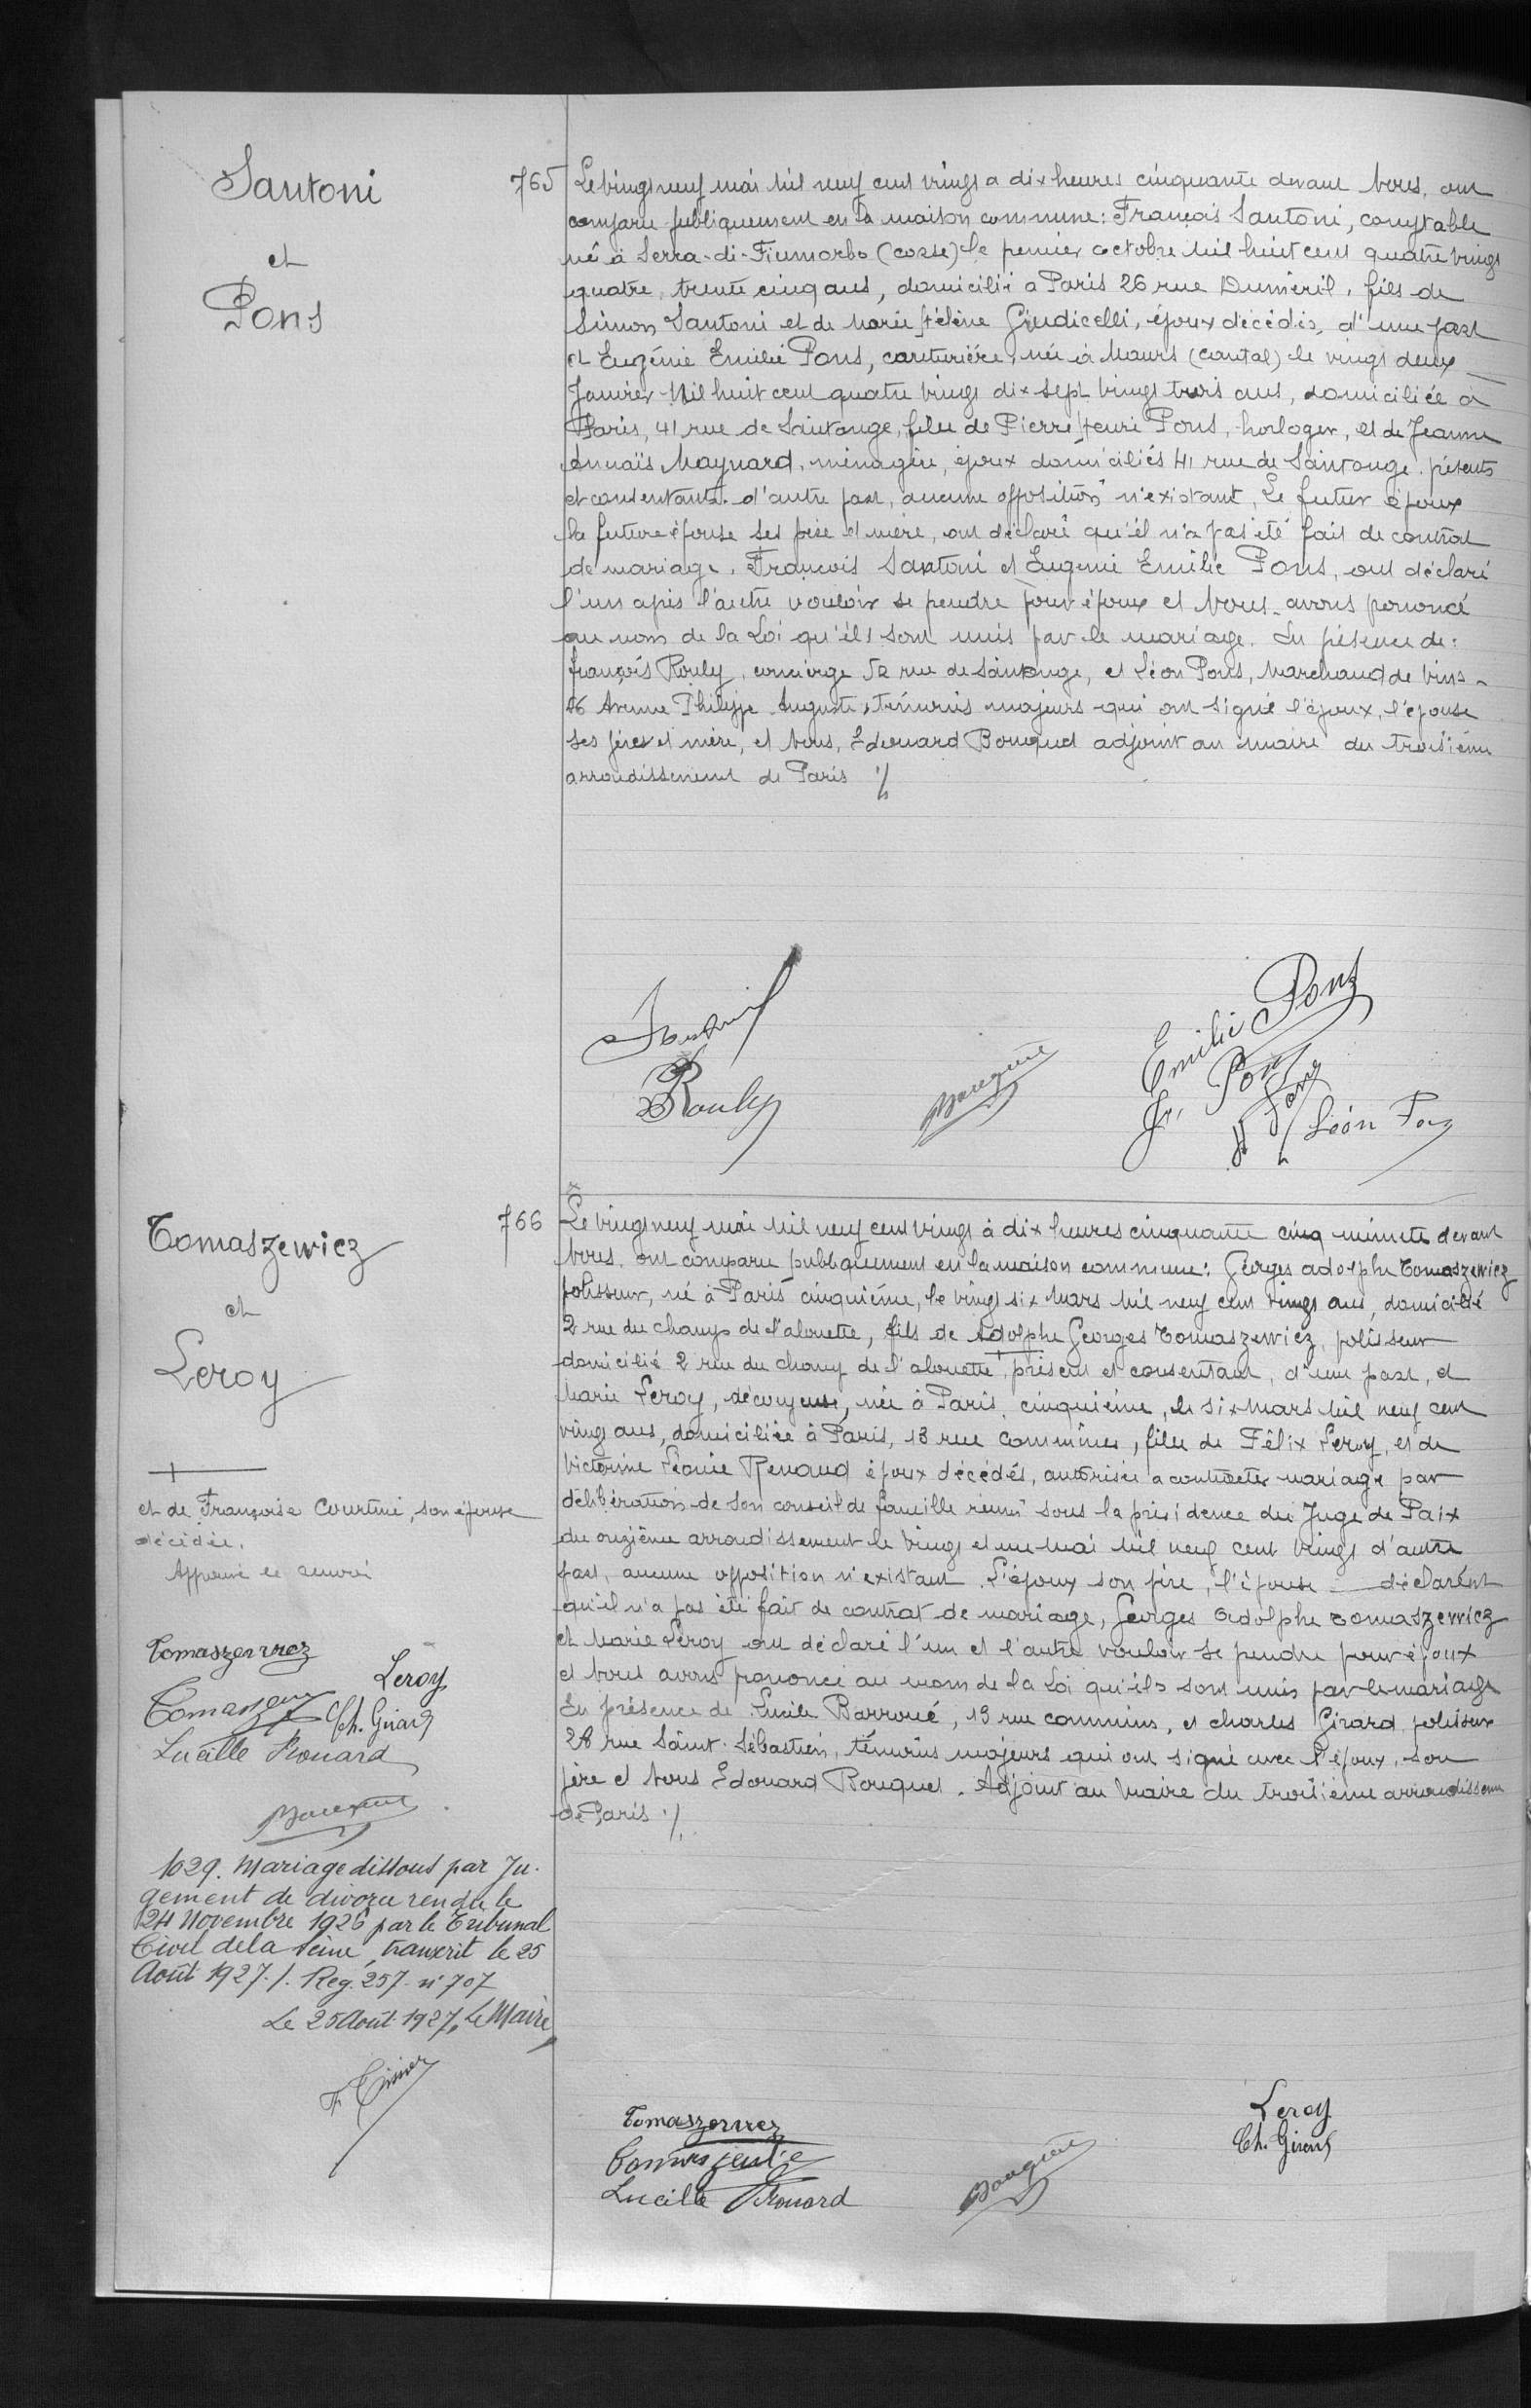Santoni 765
et
Pons
Le vingt neuf mai mil neuf cent vingt a dix heures cinquante devant Nous, ont
comparu publiquement en la maison commune: François Santoni, comptable
né à Serra-di-Fiumorbo (corse) le premier octobre mil huit cent quatre vingt
quatre, trente cinq ans, domicilié à Paris 26 rue Duméril, fils de
Simon Santoni et de Marie Hélène Grudicelli, époux décédés, d'une part
et Eugénie Emilie Pons, couturière, née à Maurs (cantal) le vingt deux
Janvier Mil huit cent quatre vingt dix sept vingt trois ans, domiciliée à
Paris, 41 rue de Saintonge, fille de Pierre Henri Pons, horloger, et de Jeanne
Anaïs Maynard, ménagère, époux domiciliés 41 rue de Saintonge, présents
et consentants. d'autre part, aucune opposition n'existant, Le futur époux
la future épouse ses père et mère, ont déclaré qu'il n'a pas été fait de contrat
de mariage. François Santoni et Eugénie Emilie Pons, ont déclaré
l'un après l'autre vouloir se prendre pour époux et Nous avons prononcé
au nom de la Loi qu'ils sont unis par le mariage. En présence de:
françois Rouly, concierge 50 rue de Saintonge, et Léon Pons, marchand de vins.
46 Avenue Philippe Auguste, témoins majeurs qui ont signé l'époux, l'épouse
ses père et mère, et Nous, Edouard Bouquet adjoint au maire du troisième
arrondissement de Paris ./.
766
Tomaszewiez
et
Leroy
Le vingt neuf mai mil neuf cent vingt à dix heures cinquante cinq minutes devant
Nous ont comparu publiquement en la maison commune: Georges adolphe Tomaszewiez
polisseur, né à Paris cinquième, le vingt six mars mil neuf cent vingt ans, domicilié
2 rue du champs de l'alouette, fils de Adolphe Georges Tomaszewiez, polisseur
domicilié 2 rue du champs de l'alouette, présent et consentant, d'une part, et
Marie Leroy, découpeuse, née à Paris, cinquième, les six mars mil neuf cent
vingt ans, domiciliée à Paris, 13 rue commius, fille de Félix Leroy, et de
Victorine Léonie Renaud époux décédés, autorisés à contracter mariage par
délibération de son conseil de famille réuni sous la présidence du Juge de Paix
du onzième arrondissement le vingt et un mai mil neuf cent vingt d'autre
part, aucune opposition n'existant. L'époux son père, l'épouse, déclarent
qu'il n'a pas été fait de contrat de mariage, Georges Adolphe Tomaszewiez
et Marie Leroy ont déclaré l'un et l'autre vouloir se prendre pour époux
et Nous avons prononcé au nom de la Loi qu'ils sont unis par le mariage
En présence de Lucile Barroué, 13 rue commius, et Charles Girard, poliseur
28 rue Saint Sébastien, témoins majeurs qui ont signé avec l'époux, son
père et Nous Edouard Bouques, Adjoint au Maire du troisième arrondissement
de Paris ./.
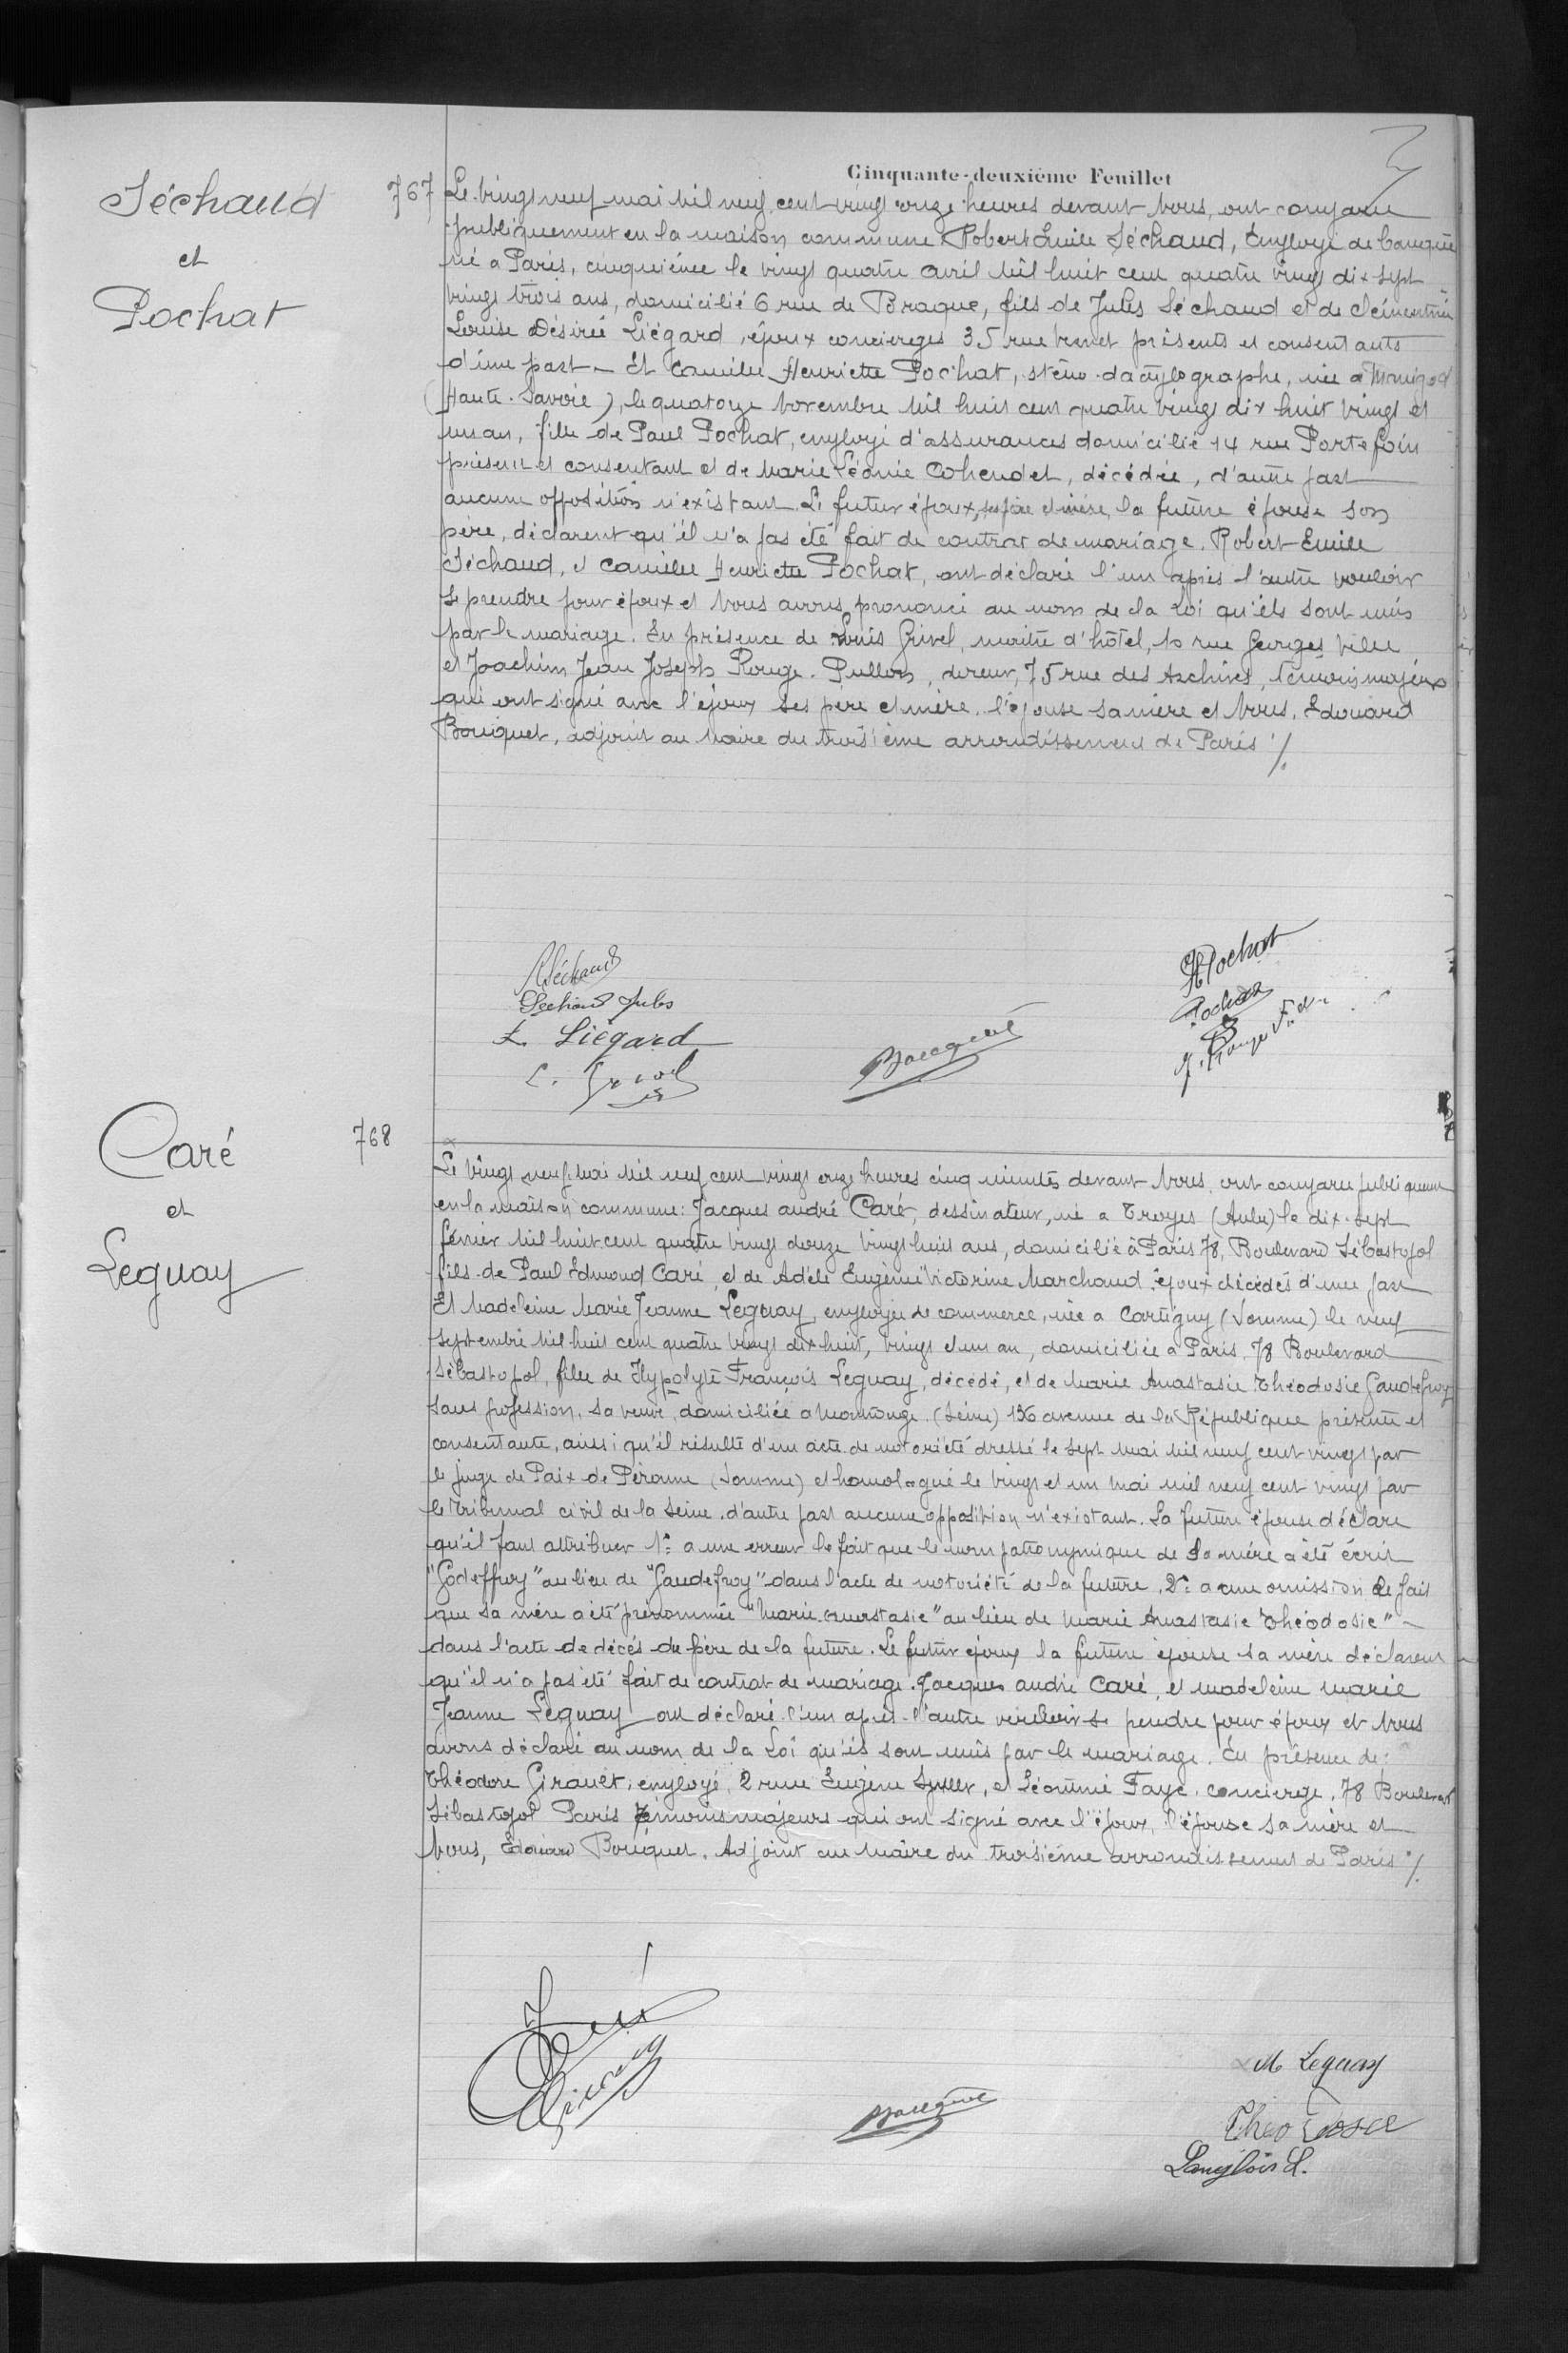Séchaud 767
et
Pochat
Le vingt neuf mai mil neuf cent vingt onze heures devant Nous, ont comparu
publiquement en la maison commune Robert Emile Séchaud, Employé de banque
né à Paris, cinquième le vingt quatre Avril mil huit cent quatre vingt dix sept
vingt trois ans, domicilié 6 rue de Braque, fils de Jules Séchaud et de Clémentine
Louise Désirée Liégard, époux concierges 35 rue Vernet présents et consentants
d'une part-Et Camille Henriette Pochat, sténo dactylographe, née à Manigod
(Haute-Savoie), le quatorze novembre mil huit cent quatre vingt dix huit vingt et
un an, fille de Paul Pochat, employé d'assurances domicilié 14 rue Portefoin
présents et consentants et de Marie Léonie Cohendet, décédée, d'autre part
aucune opposition n'existant. Le futur époux, ses père et mère, la future épouse son
père, déclarent qu'il n'a pas été fait de contrat de mariage. Robert Emile
Séchaud, et Camille Henriette Pochat, ont déclaré l'un après l'autre vouloir
se prendre pour époux et Nous avons prononcé au nom de la Loi qu'ils sont unis
par le mariage. En présence de Louis Grivel, maitre d'hôtel, 10 rue Georges Ville
et Joachim Jean Joseph Rouge Pullons, cireur, 75 rue des Archives, témoins majeurs
qui ont signé avec l'époux des père et mère l'épouse sa mère et Nous, Edouard
Bouquet, adjoint au Maire du troisième arrondissement de Paris.
Carré 768
et
Leguay
Le vingt neuf mai mil neuf cent vingt onze heures cinq minutes devant Nous ont comparu publiquement
en la Maison commune: Jacques André Caré, dessinateur, né à Troyes (Aube) le dix-sept
février mil huit cent quatre vingt douze vingt huit ans, domicilié à Paris 78, Boulevard Sébastopol
fils de Paul Edmond Caré, et de Adèle Eugénie Victorine Marchand époux décédés d'une part
Et Madeleine Marie Jeanne Leguay, employée de commerce, née Cartigny (Somme) le vingt
septembre mil huit cent quatre vingt dix huit, vingt et un an, domiciliée à Paris, 78 Boulevard
Sébastopol, fille de Hypolyte François Leguay, décédé, et de Marie Anastasie Théodosie Gaudefroy
sans profession, sa veuve, domiciliée à Montrouge (Seine) 136 avenue de la République présente et
consentante, ainsi qu'il résulte d'un acte de notoriété dressé le sept mai mil neuf cent vingt par
le juge de Paix de Péronne (Somme) et homologué le vingt et un mai mil neuf cent vingt par
le Tribunal civil de la Seine, d'autre part aucune opposition n'existant. La future épouse déclare
qu'il faut attribuer 1°: a une erreur le fait que le nom patronymique de sa mère a été écrit
"Godeffroy" au lieu de "Gaudefroy" dans l'acte de notoriété de la future, 2°: aucune omission de fait
que sa mère a été prénommée "Marie Anastasie" au lieu de Marie Anastasie Théodosie" -
dans l'acte de décès du frère de la future. Le futur époux la future épouse sa mère déclarent
qu'il n'a pas été fait de contrat de mariage. Jacques André Caré, et Madeleine Marie
Jeanne Leguay ont déclaré l'un après l'autre vouloir se prendre pour époux et Nous
avons déclaré au nom de la Loi qu'ils sont unis par le mariage. En présence de:
Théodore Girauet, employé, 2 rue Eugène Spuller, et Léontine Fayet, concierge, 78 Boulevard
Sébastopol Paris, témoins majeurs qui ont signé avec l'époux, l'épouse sa mère et
Nous, Edouard Bouquet, Adjoint au Maire du troisième arrondissement de Paris ./.